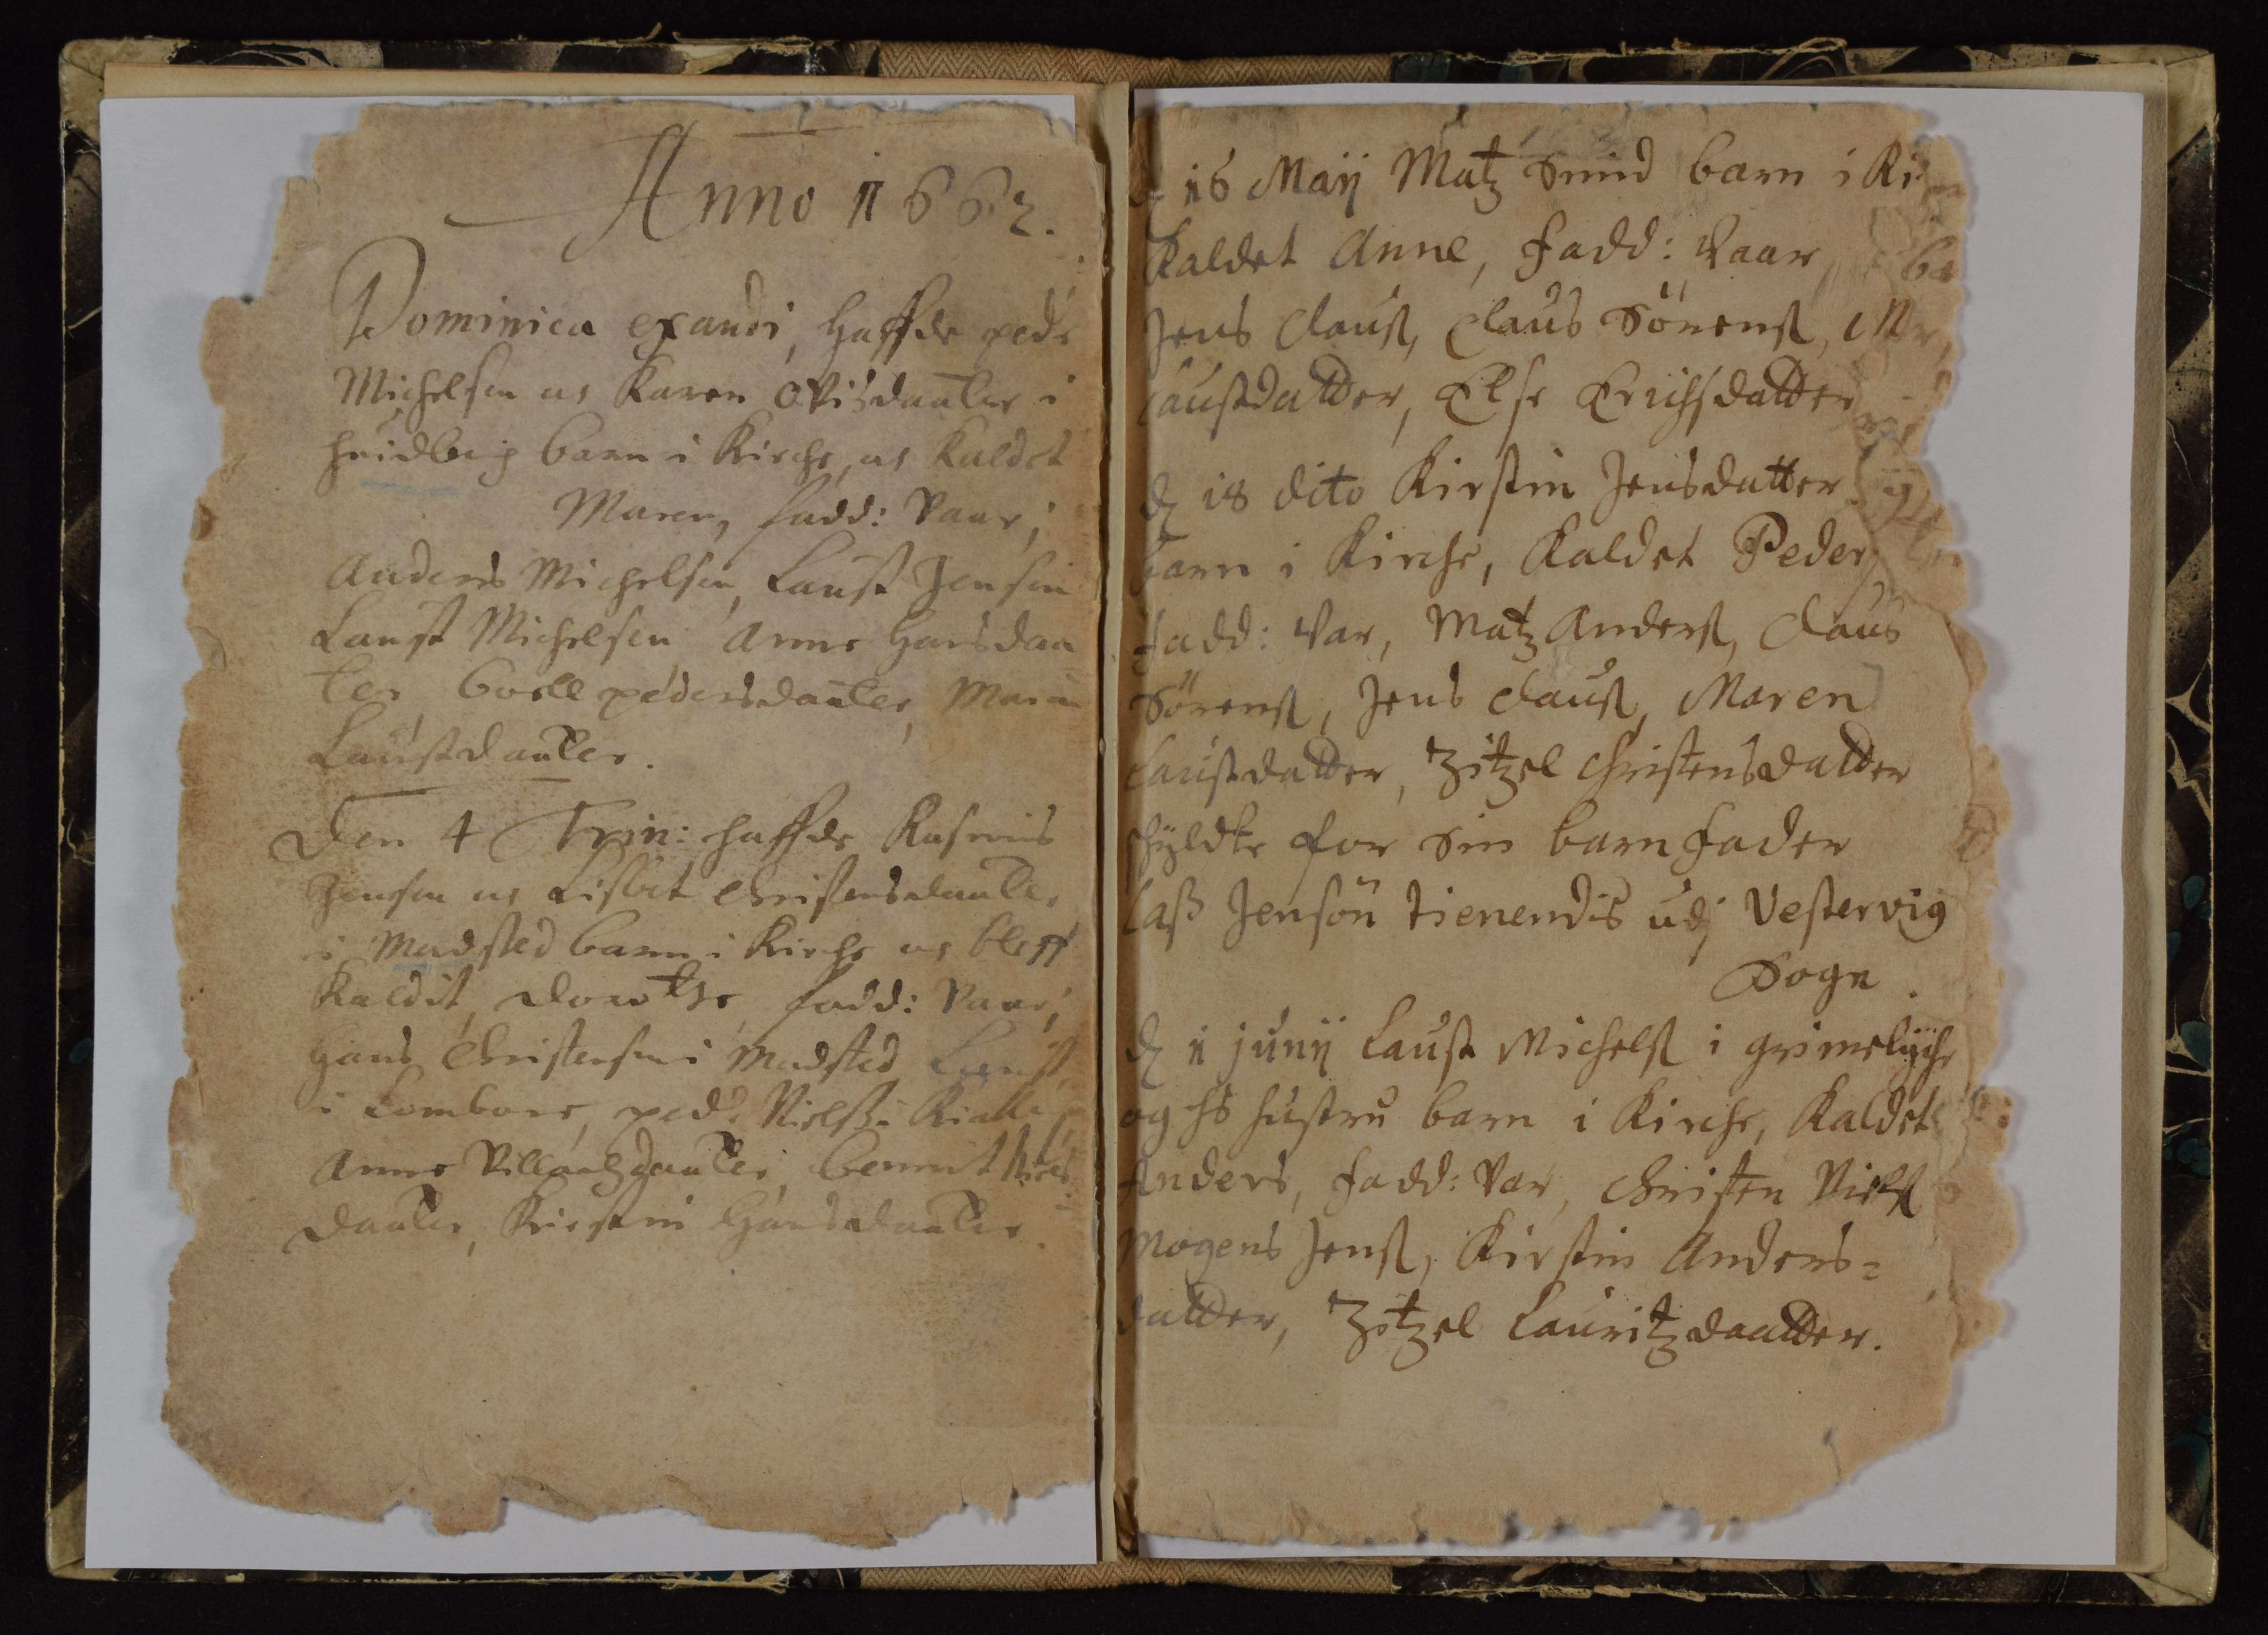Anno 1662.
Dominica exaudi Haffde pedr
Michelsen och Karen Ovisdaater i
Hvidberg barn i Kirche, och kaldet
Maren Fadd: vaar
Anders Michelsen Laust Jensen
Laust Michelsøn Anne Hansdaa
ter Boell pedersdauter, Maren
Laustdauter
Den 4 Trin: haffde Rasmus
Jensen och Lisbet Christensdaater
i Madsted barn i Kiche och bleff
kaldit Dorthe fadd: vaar
Hans Christensen i Madsted Laust
i lombone pedr Niels i  Kiallerup
Anne Villadzdaater Bennit Niels
daater, Kirsten Hansdatter
16 Maj Matz Smid barn i Ri  
kaldet Anne, Fadd: vaar
Jens Claus, Claus Sørens, ##  
 laustdatter Else Erichsdatter
d 18 dito Kirstin Jensdatter
barn i Kirche, kaldet Peder
Fadd: var, Matz Anders Claus
Sørens, Jens Claus, Maren
Laustdatter, Zitzel Christensdatter
skyldte for sin barn Fader
Lass Jensøn tienendis udi Vestervig
Sogn
d 1 Junij Llaust Michels i Grimslyche
og hs hustru barn i Kirche, kaldet
Anders, Fadd: var Christen Niels
Mogens Jens, Kirstin Anders¬
datter, Zitzel Lauritz dautter.
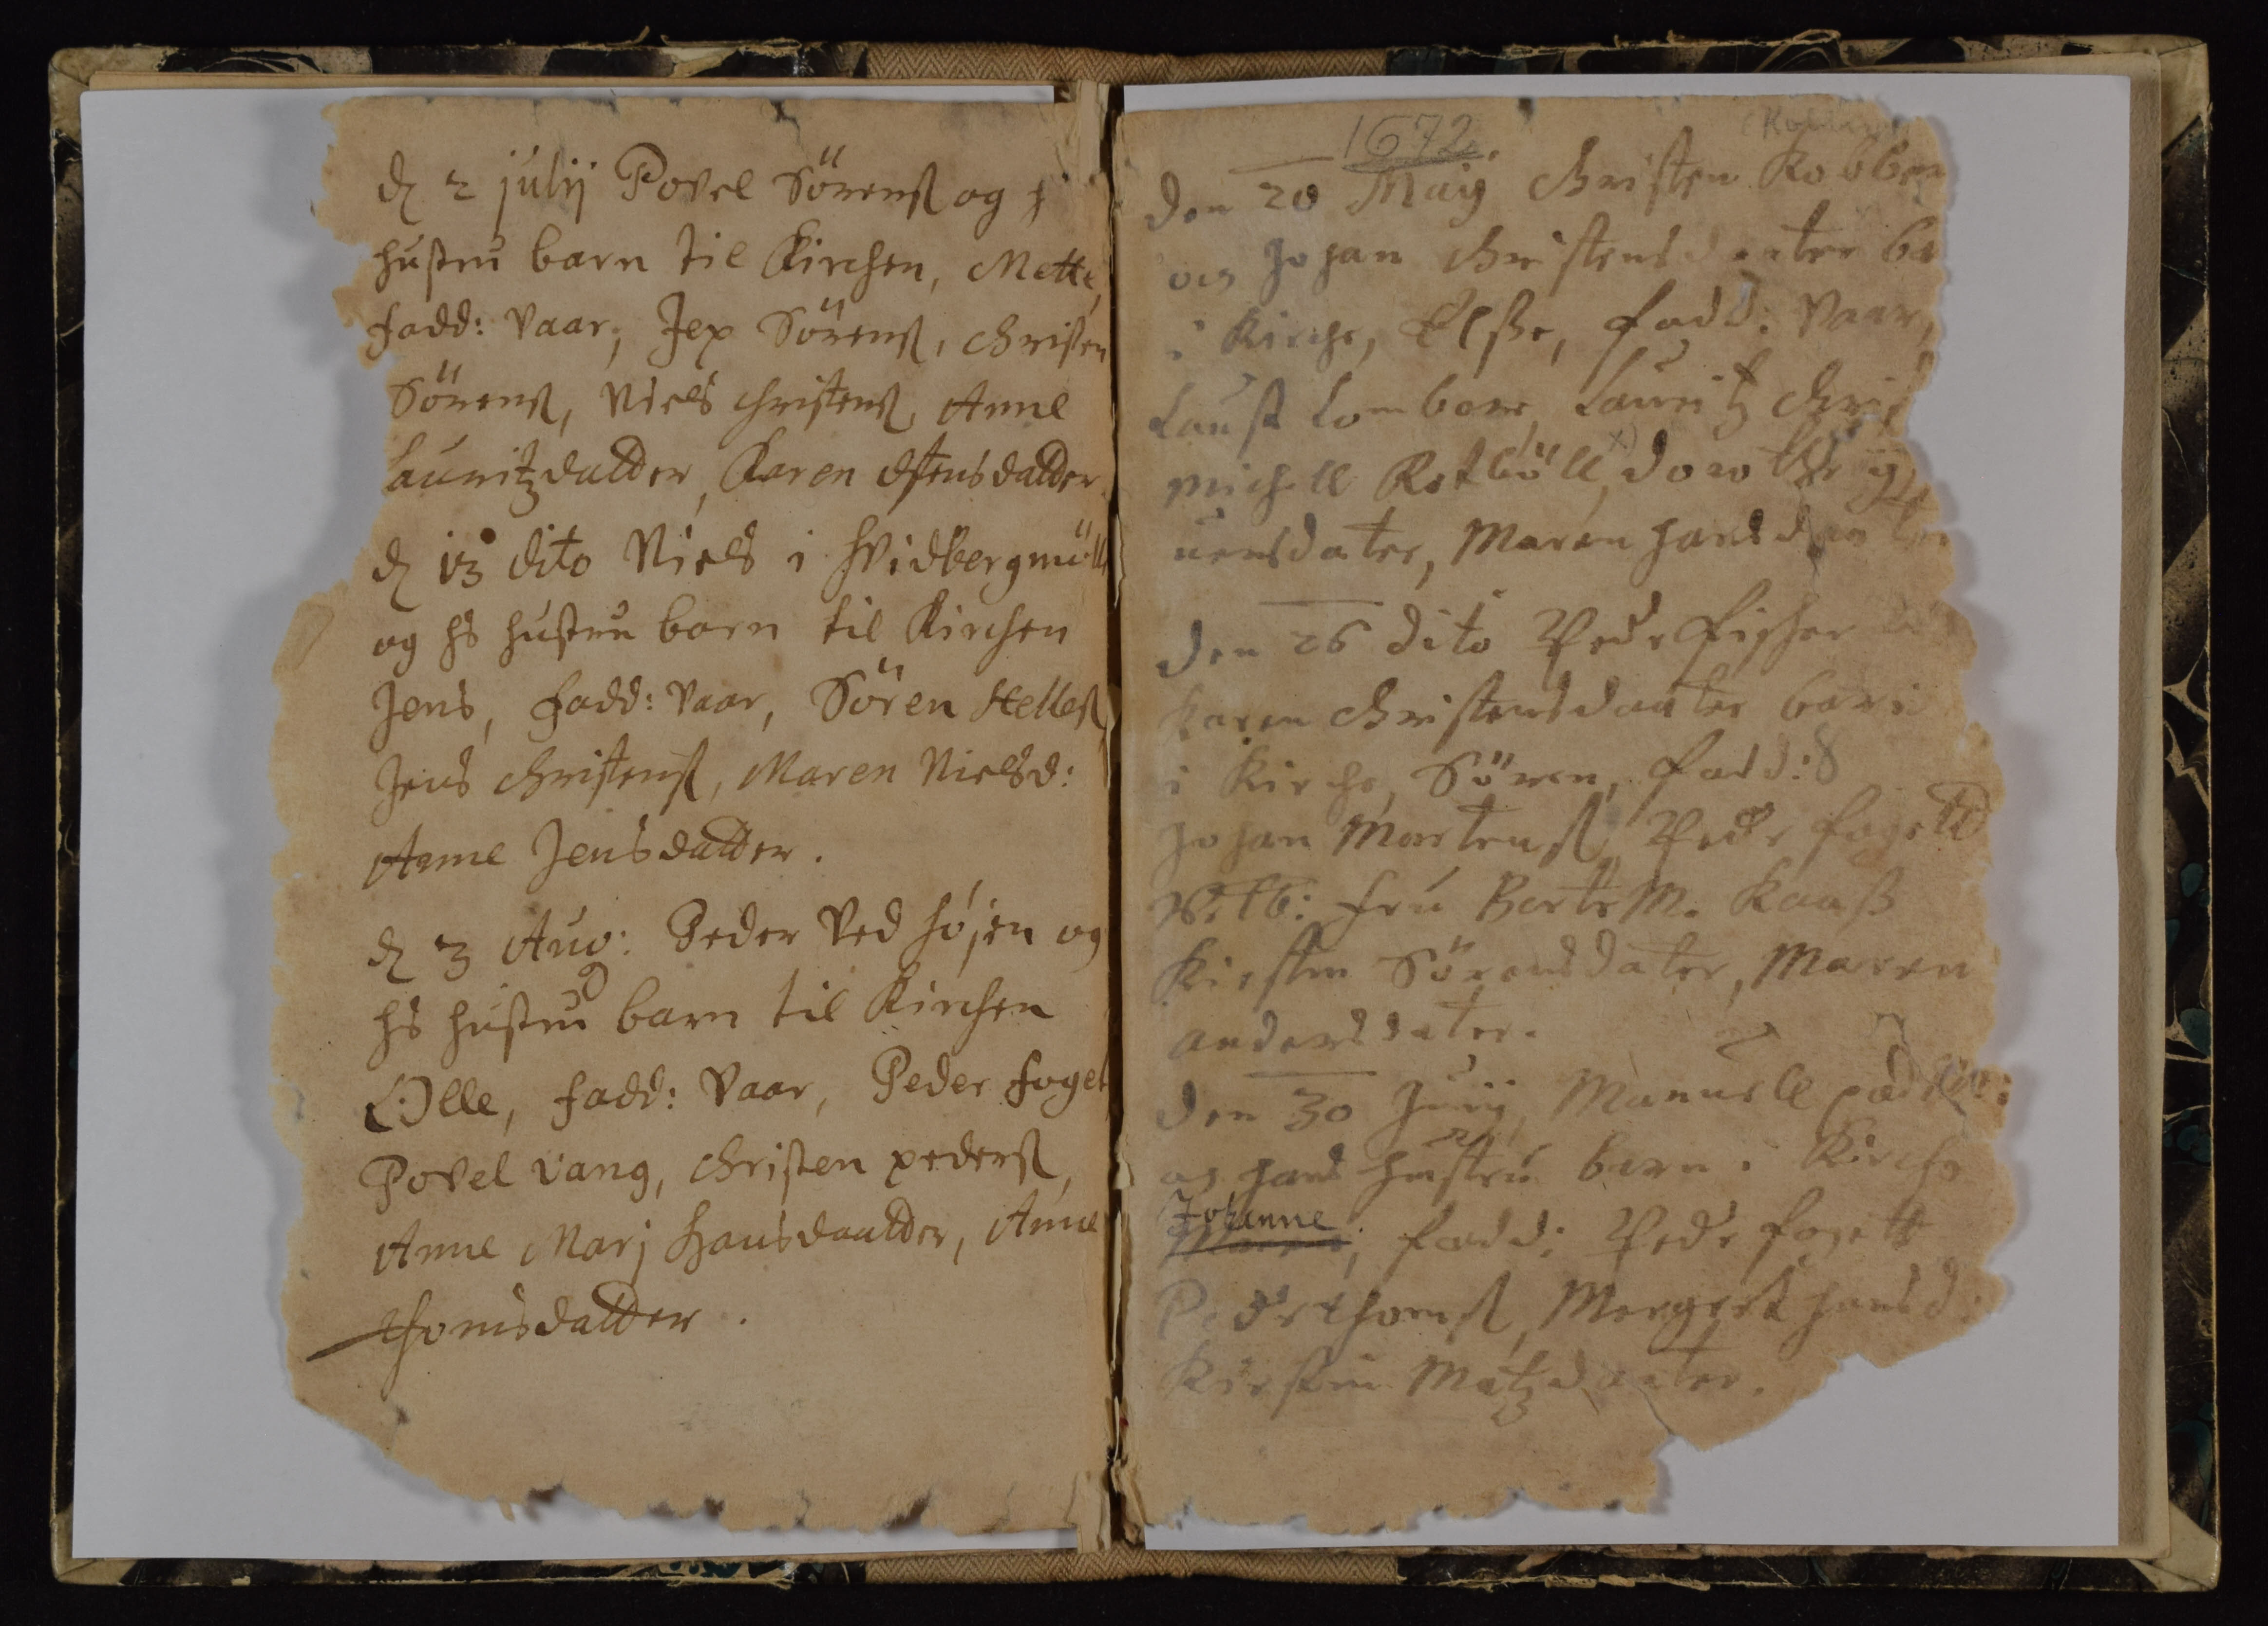d 2 Julij Povel Sørens og h
hustru barn til Kirchen, Mette
fadd: Vaar; Jep Sørens, Christen
Sørens, Niels Christens. Anne
Lauritzdatter, Karen ##stensdatter
d 13 dito Niels i Hvidberg mølle
og hs hustru born til Kirchen
Jens, Fadd: vaar, Søren Helles
Jens Christens, Maren Nielsd:
Anne Jensdatter.
d 3 Aug: Peder ved Højen og
hs hustru barn til Kirchen
Olle, Fadde vaar, Peder Foget
Povel Vang, Christen peders
Anne Marj Hansdautter, Anne
Thomsdatter
Den 20 Maij Christn Kobb##
och Johan Christensdaater ba  
i Kirche Elsse, Fadd. vaar,
Laust Lombone Lauritz Chris
Michell Rotbøll, Doro ##
uensdater, Maren Hansdaater
den 26 dito Pedr Fisher och
Karen Christensdauter barn
i Kirche Søren, Fadd: ##
Johan Mortens Pedr fogett
Velb: fru Berte M. Kaasa
Kirsten Sørensdater, Maren
Andersdater.
Den 30 Junij Manuell pæd##
och hans hustru barn i Kirche
Johanne; Fadd: Peder fogett
Peder Thoms Margrete Hansd:
Kirstin Matzdaater.